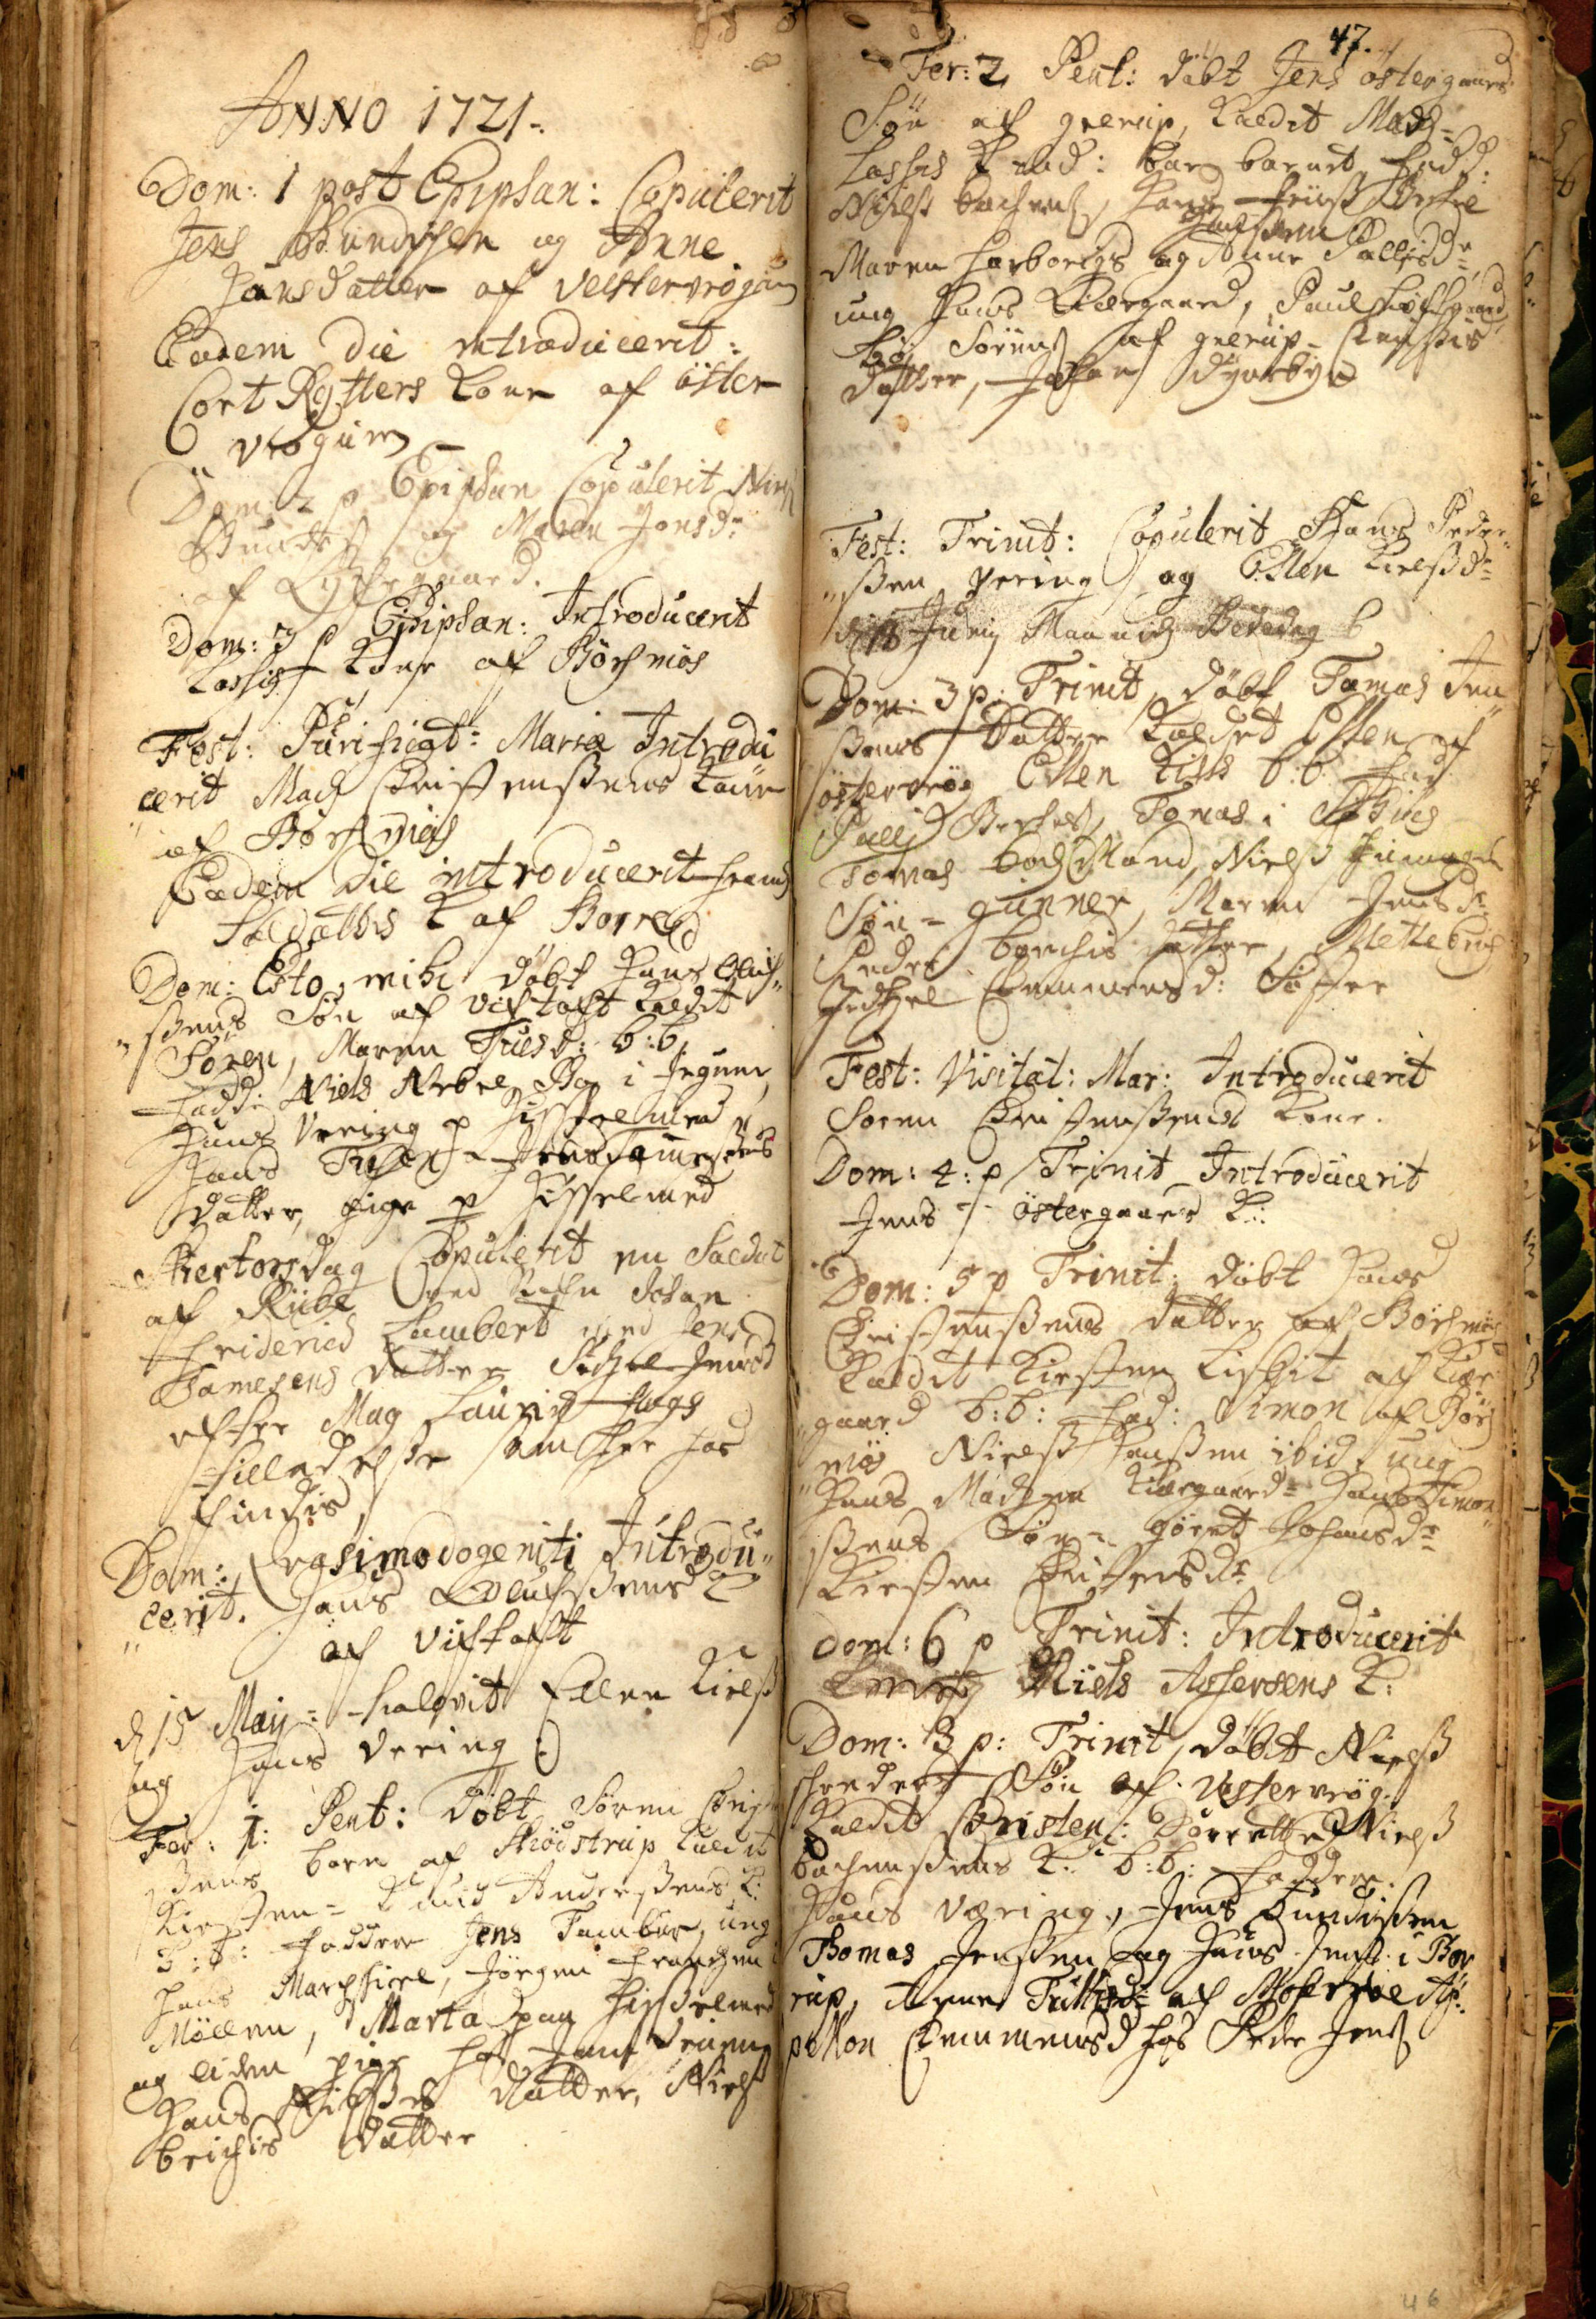Anno 1721:
Dom: 1 post Epiphan: Copulerit
Jens Bundisen og Anne
Hansdatter af Vestervrøgum
Eodem Die introducerit
Cort Rytters Kone af Øster¬
vrøgum
Dom: 2 p Epiphan Copulerit Niels
Bundisen og Maren Jonsdr
af Lychegaard.
Dom: 3 p Epiphan:  Introducerit
Lassis Kone af Børsmøs
Fest: Purificat: Mariæ Introdu¬
cerit Madz Christensens Kone
af Børsmøs
Eodem Die introducerit Frandz
Soldats K af Borre
Dom: Esto Mihi døbt Hans Oluf¬
sens Søn af Viftoft kaldit
Søren, Maren Tuesd: b:b:
Fadd: Niels Nebel Boj i Jegum
Hans Væring p Hisselmed
Hans Tr## Jens Tommesøns
Datter pige p Hisselmed
Skiertorsdag Copulerit en Soldat
af Riibe ved Nafn Johan
Friderich Lambert med Jens
Tamesens Datter Sidzel Jensd
efter Mag Lauridz Fogs
Tilladelse som her hos
findis
Dom: Qvasimodogeniti Introdu¬
cerit Hans Olufsens K
af Viftoft
D 15 Maij= trolovit Ellen Kiels
og Hans Vering
Fer: 1: Pent: døbt Søren Christen
sens Barn af Skiødstrup kaldit
Kirsten= Knud Andersens K:
b: b: Faddere Jens Tambur, ung
Hans Marchschiel, Jørgen Frandzen i
Møllen, Marta paa Hisselmed
og liden pige hos Jens Vrøgum
Hans Nissens Datter, Niels
Brichis Datter
47
46
Fer: 2 Pent: døbt Jens Østergaars
Søn af Grerup. kaldit Madz
Lassis K ibid: bar barnet. Fadd
Niels Bachens Hans Friis Bertel
Hansen
Maren Harborigs og Anne Palljsdr
ung Hans Kiærgaard, Poul Løftgaard
Boj Sørens af Grerup Clausis
Datter, Johan Dyrebye
Fest: Trinit: Copulerit Hans Peder¬
sen Vering og Ellen Kielsdr
d 18 Junij Maaneds Bededag
Dom: 3 p: Trinit døbt Tomas Jen¬
sens Datter kaldet Ellen af
Østervrøg Ellen Kiels b:b: Fad
Pallj Bertels, Tomas i Søhuus
Tomas Bodz Mand, Niels Schumagers
Søn= Gunner, Maren Jensdr
Peder Borchis Datter, Mette Brich
##idzel Clemmensd: Søster
Fest: Visitat: Mar: Introducerit
Søren Christensens Kone.
Dom: 4: p Trinit Introducerit
Jens Østergaars K:
Dom: 5 p Trinit: døbt Hans
Christensens Datter af Børsmøs
kaldit Kirsten Lisbet af Kiær¬
gaard b:b: Fad: Simon af Børs
møs Niels Hansen ibid: ung
Hans Madzen Kiærgaard= Hans Simon¬
sens Søn= Gøret Johansdr
Kirsten Christensdr
Dom: 6 p Trinit: Introducerit
L## Niels Assersens K:
Dom: 8 p: Trinit. døbt Niels
Schreders Søn af Vestervrøg.
kaldit Christen: Dorrette Niels
Bachensens K: b:b: Faddere:
Hans Væring, Jens Bundisen,
Thomas Jensen og Hans Jens i Bor¬
rup, Anne Tullesdr af Mosevroe, Ap¬
pelon Clemmensd hos Peder Jens
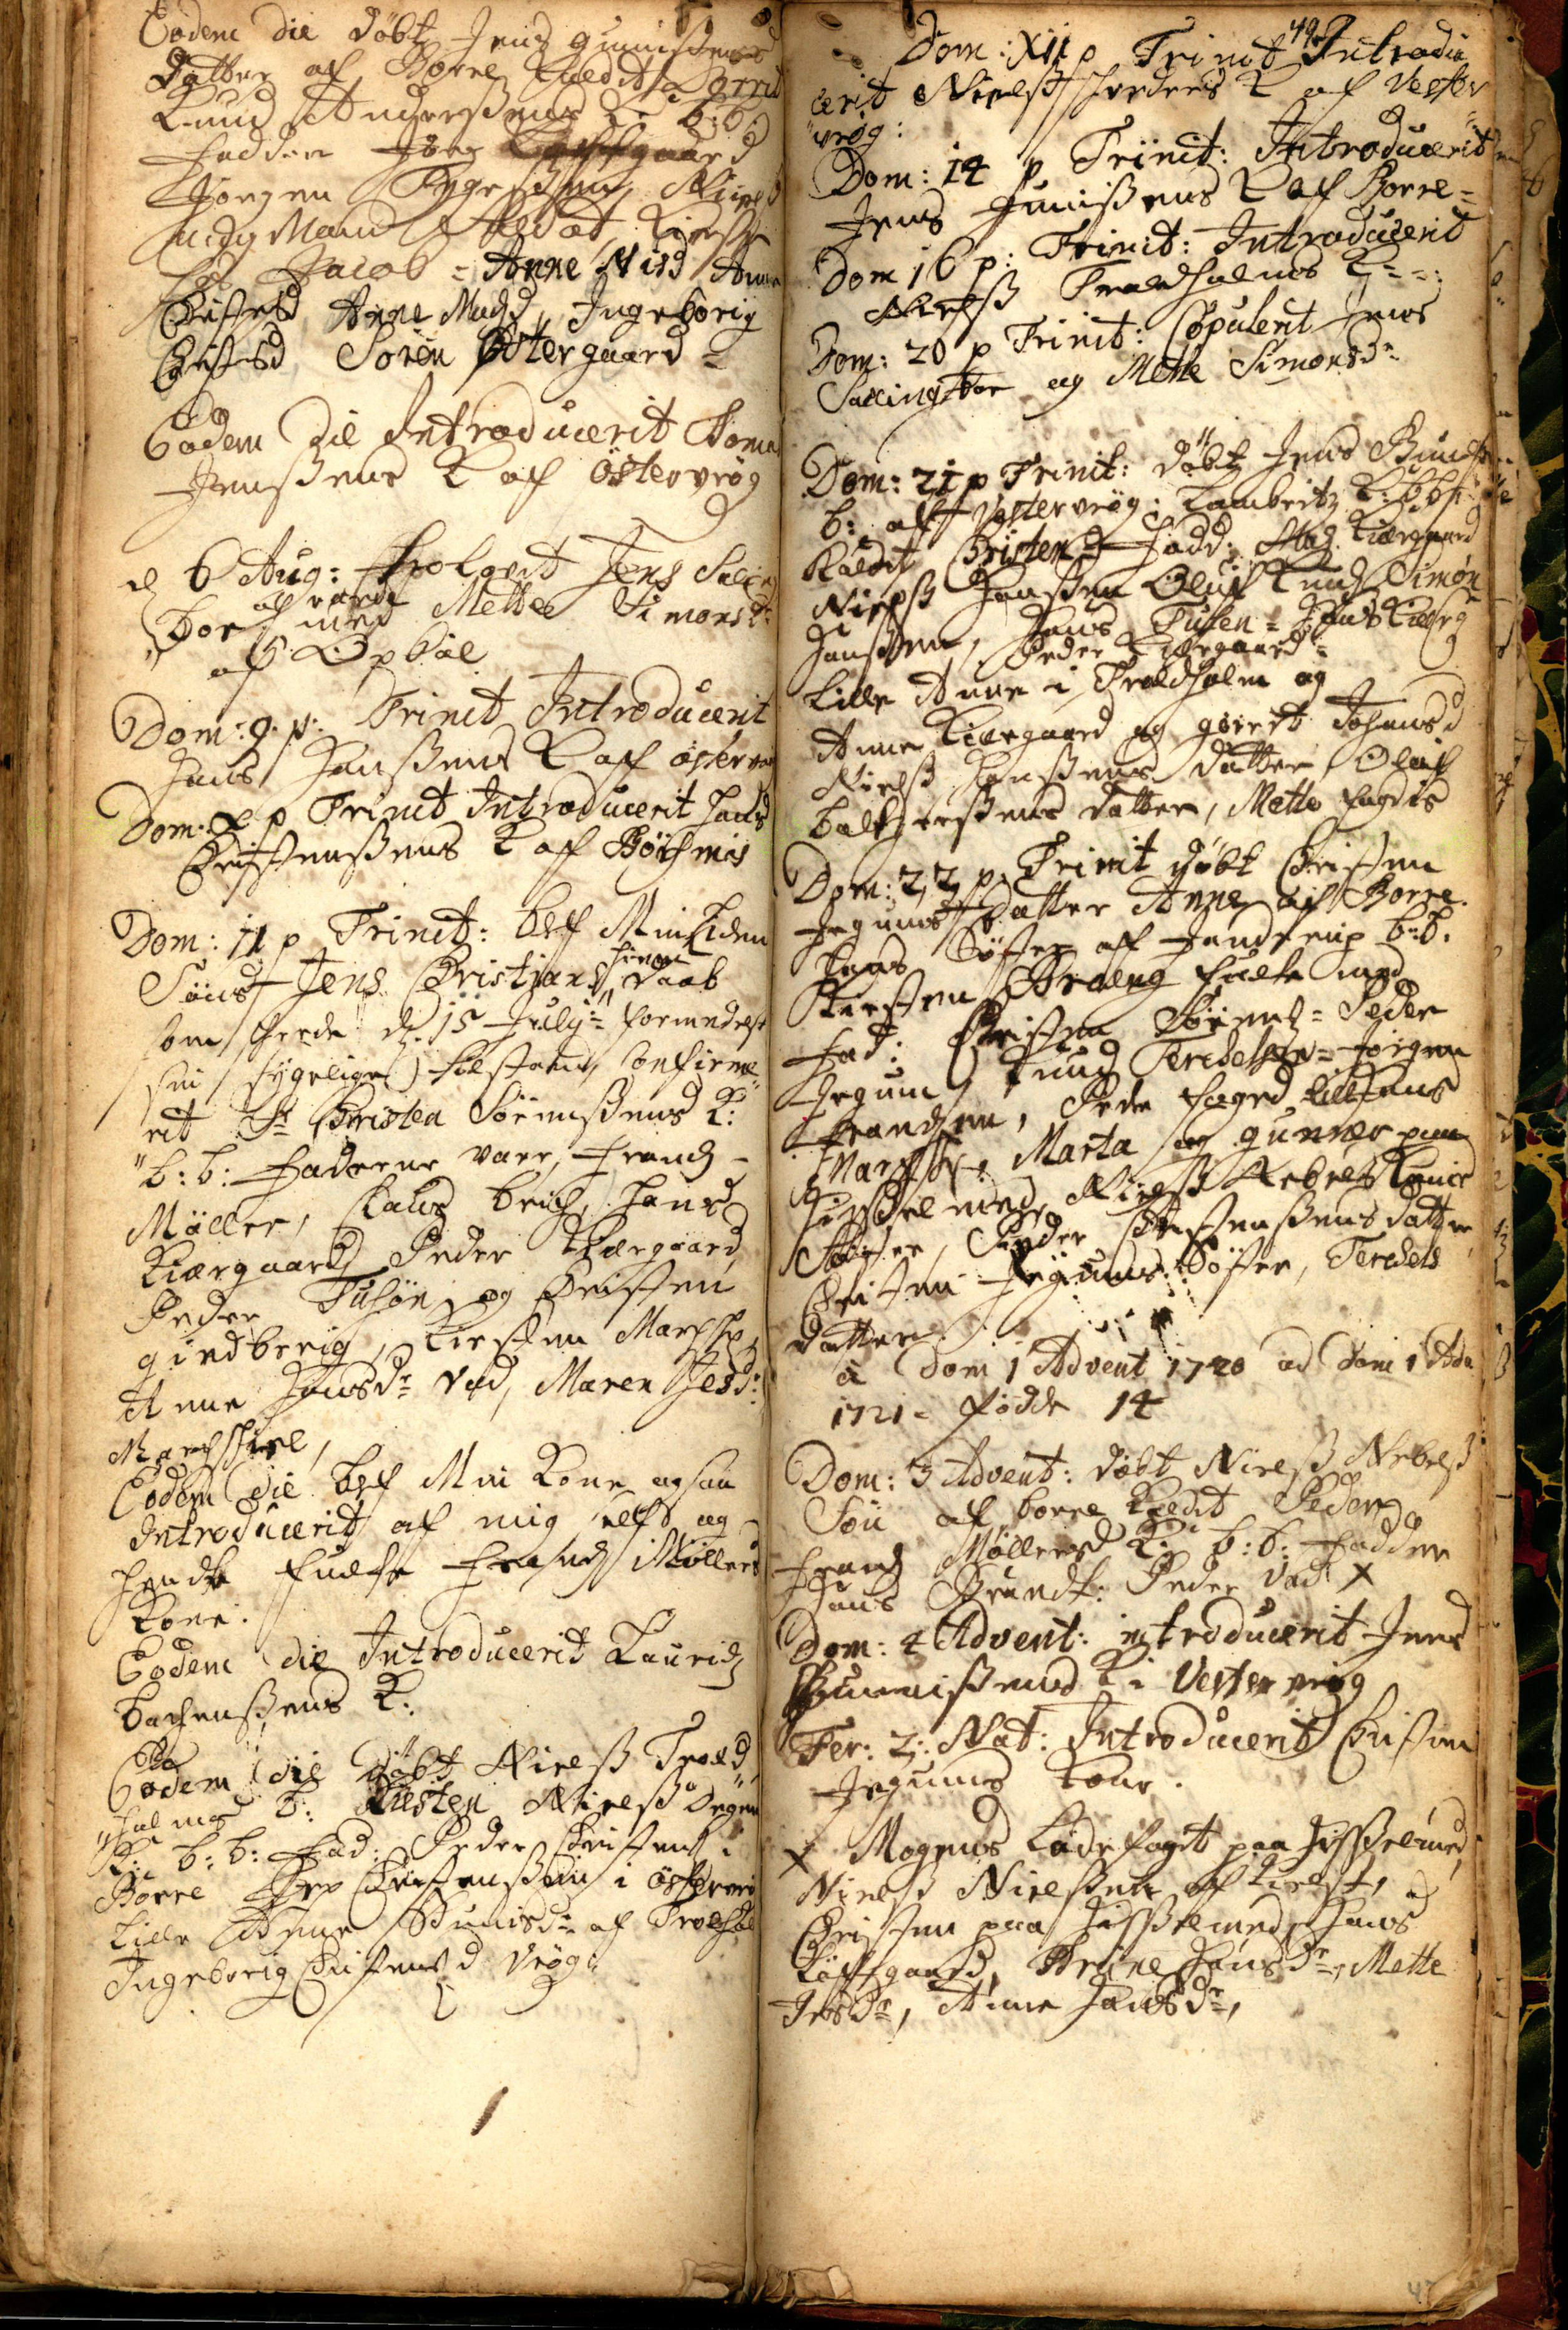Eoedem Die døbt Jens Gunnisens
Datter af Borre kaldit Dorrit
Knud Andersens K: b:b:
Faddere Jørg Løftgaard
Jørgen Tygesen Niels
ung Mand Soldat, Kirsten
hos Jacob= Anne Nisd, Anne
Christensd Anne Madzd, Ingeborig
Christensd, Søren Østergaard=
Eodem Die Introducerit Thomas
Jensens K af Østervrøg
D 6 Aug: trolovet Jens Salling
af Varde
boe med Mette Simonsdr
af Oxbø
Dom: 9. p:Trinit Introducerit
Hans Hansens K af Østervrøg
Dom: X p Trinit Introducerit Hans
Christensens K af Børsmøs
Dom: 11 p Trinit: blef Min Liden
hiemme
Søns Jens Christians daab
som scheede d.15 Julij formedelst
sin sygelige Tilstand, Confirme¬
rit. Sr Christen Sørensens K:
b:b: Faderne vare, Frandz
Møller, Claus Brich, Hans
Kiærgaard, Peder Kiærgaard
Peder Tusøn og Christen
Giedberig, Kirsten Marchsch
Anne Hansdr  Vad, Maren Jesdr
Marchschiel
Eodem Die blef Min Kone ogsaa
Introducerit af mig self og
hende fulte Frandz Møllers
Kone.
Eodem Die Introducerit Lauridz
Bachensens K:
Eodem Die Døbt Niels Trold¬
holms B: Kiesten Niels Degns
K: b:b: Fad: Peder Christens i
Borre Jep Christensen i Østervrø
Lille Anne Bundisdr af Troldhol
Ingeborig Christensd af Vrøg:
49
Dom: XII p Trinit Introdu
cerit Niels Schreders K af Vester
vrøg:
Dom:14 p Trinit: Introducerit
Jens Gunnisens K af Borre=
Dom 16 p: Trinit: Introducerit
Niels Troldholms K==:
Dom: 20 p Trinit: Copulerit Jens
Sallingboe og Mette Simonsdr
Dom: 21 p Trinit: døbt Jens Bundisens
B: af Vestervrøg: Lambertz K: bb
kaldit Christen Fadd: Madz Kiærgaard
Niels Hansen Oluf Knudzen Simon
Hansen, Hans Tusen= Hans Kiærg
Peder Kiærgaards ##
Lille Anne i Troldholm og
Anne Kiærgaard og Gørrit Johansd
Niels Hansens Datter, Oluf
Baltzersens Datter, Mette Fogdis
Dom: 22 p: Trinit døbt Christen
Jegums Datter Anne af Borre
Hans Søster af Janderup b:b:
Kirsten Broeng fulte med
Fad: Christen Sørens= Peder
Jegum, Knud Terchelsen= Jørgen
Frandzen, Peder Foged, Lille Hans
Marchsch, Marta og Gunner paa
Hisselmed, Niels Nebels Kones
Søster, Peder Christensens Datter,
Christen Jegums Søster, Terchels
Datter
## Dom 1 Advent 1720 ad Dom 1 Adv
1721= fødde 14
Dom: 3 Advent: døbt Niels Nebels
Søn af Borre kaldit Peder
Frandz Møllers K: b:b: Faddere
Hans Brandt: Peder Vad, X
Dom: 4 Advent: introducerit Jens
Bunnisens K i Vestervrøg
Fer: 2: Nat: Introducerit Christen
Jegums Kone
X Mogens Ladefogit paa Hiselmed
Niels Nielsen af Kielst,
Christen paa Hisselmed, Hans
Løftgaard, Thrine Hansdr, Mette
Jesrdr, Anne Hansdr,